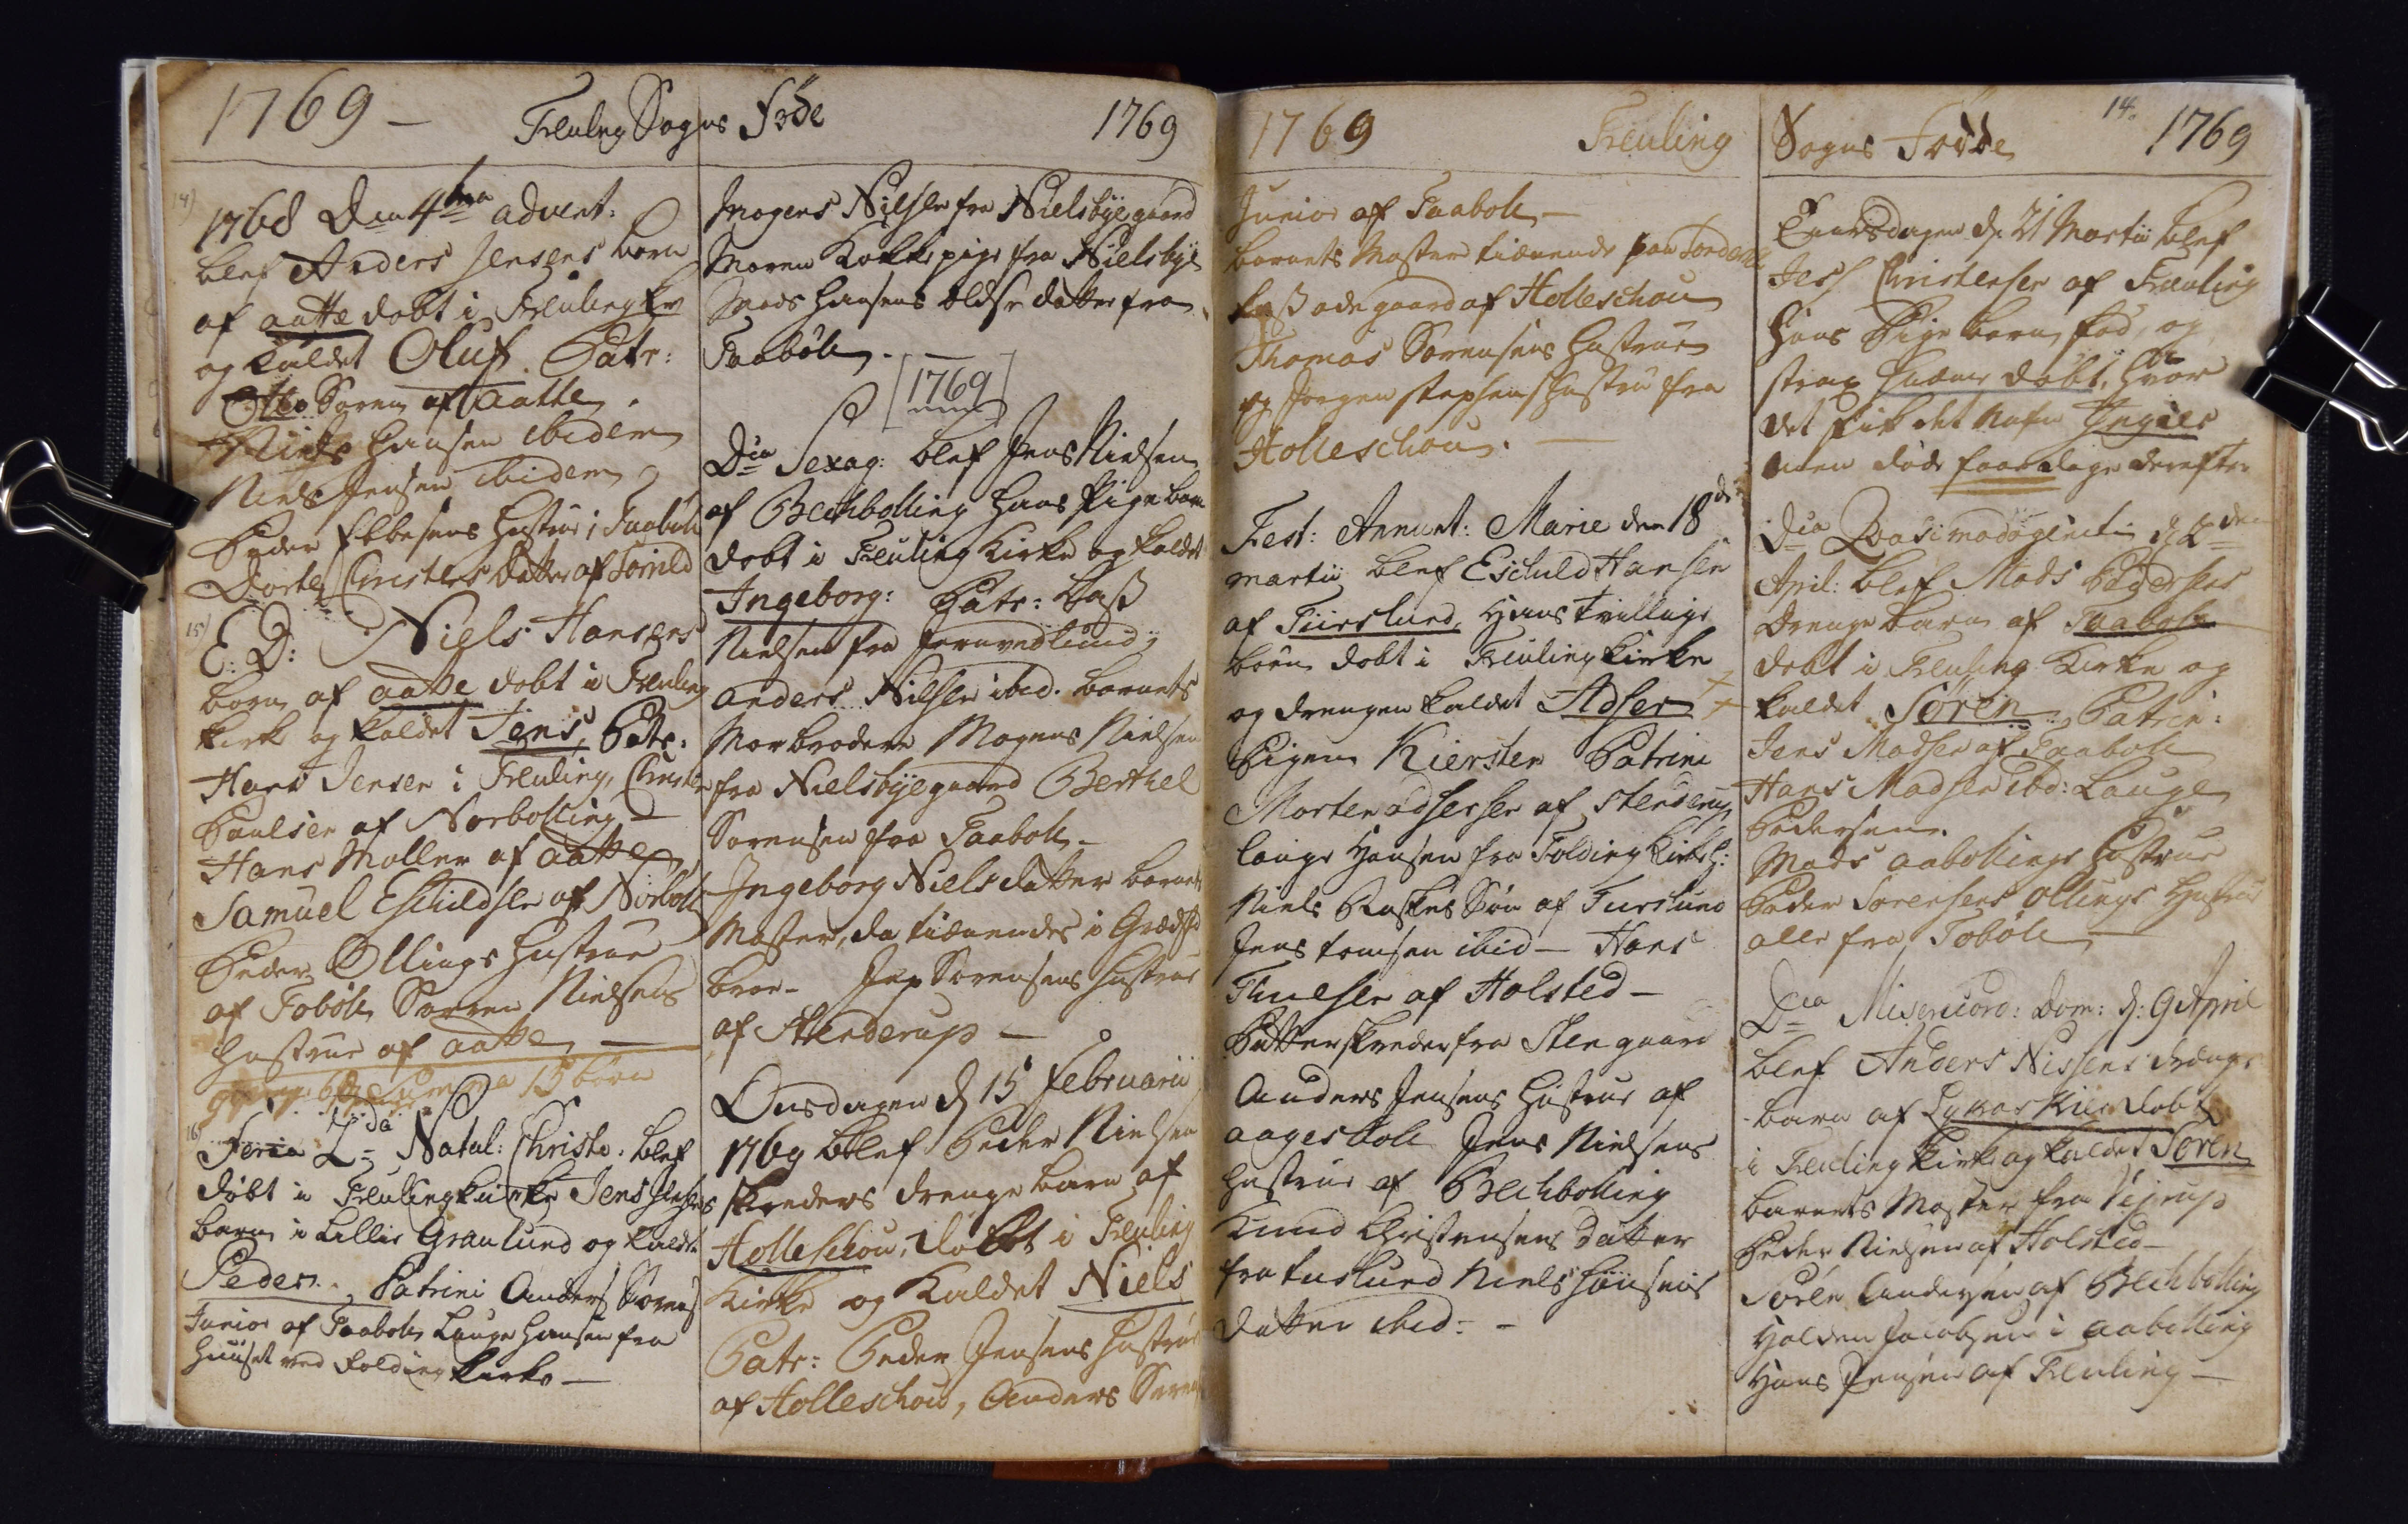1769
Feulings Sogns Føde
14)
1768 Dca 4tia Advent:
blev Anders Jensens barn
af Aatte døbt i Feuling Kr
og kaldet Oluf. Patr:
Otte Soren af Aatte
Niels Hansen ibidem
Niels Jensen ibidem
Peder Ebbesens Hustrue i Taabøll
Dorte Christens Datter af Sorrild
15
E: D: Niels Hansens
Barn af Aatte døbt i Feuling
Kirk og kaldet Jens Patr:
Hans Jensen i Feuling, Christen
Poulsen af Norbolling.
Hans Moller af Aatte
Samuel Eschildsen af Nørbolling
Peder Ollings Hustrue
af Tobøll Sorren Nielsens
Hustrue af Aatte
9 piger 6 drenge Summa 15 Børn
16)
Feria 2da Natal: Christi blef
døbt i Feuling Kirke Jens Jensens
barn i Lillie Graulund og kaldet
Peder. Patrini Anders Sørens
Junior af Taaboll Lauge Hansen fra
Huuset ved Folding Kirke -
1769
Mogens Nielsen og Nielsbyegaard
Maren Kokkepige fra Nielsbye
Mads Hansens ældse datter fra
Taabøll
1769
Dca Sexag: blef Jens Nielsen
af Bechbolling Hans Pige Barn
døbt i Feuling Kirke og kaldet
Ingeborg: Pater: Lass
Nielsen fra Jernvedlund
Anders Nielsen ibid: Barnets
Mor Broder Mogens Nielsen
fra Nielsbyegaard Berthel
Sorensen fra Taaboll
Ingeborg Niels datter barnets
Moster, da tienende i Grædsted
Broe. Jep Sørensens Hustrue
af Stenderup
Onsdagen d 15 Februarii
1769. blef Peder Nielsen
Skreders drenge Barn af
Holleschou,  døbt i Feuling
Kirke og kaldet Niels
Patr: Peder Jensens Hustrue
af Holleschou, Anders Sørens
1769
Feuling Sogns Fodde
Junior af Taaboll
Barnets Moster tienende paa ##
Lass odegaard af Holleschou
Thomas Sørensens Hustrue
og Jørgen Stephens Hustrue fra
Holleschou
Fest: Annunt: Marie den 18de
martii blef Eschild Hansen
af Tiirslund, Hans Tvillige
Børn dobt i Feuling Kirke
og Drengen kaldet Adser
Pigen Kiersten Patrini
Morten Adsersen af Stenderup
Lauge Hansen fra Folding Kirkeh:
Niels Raskes Søn af Tiirslund
Jens Tomsen ibid - Hans
Thuesen af Holsted -
P##er skredder fra Stengaard
Anders Jensens Hustrue af
aagesboll Jens Nielsens
Hustrue af Bechbolling
Knud Christensens Datter
fra tiislund Niels Hansens
datter ibid:
5
1769
Tiirsdagen d 21 Martii Blef
Jes Christensen af Feuling
hans Pige Barn fød, og
strax hieme døbt, hvor
det fik det Nafn Jngies
men døde faa##lige derefter
Dca Qvasimodogeniti d: 2den
April: Blef Mads Pedersens
Drenge Barn af Taaboll
døbt i Feuling Kirke og
kaldet Søren. Patrin:
Jens Madsen af Taaboll
Hans Madsen ibd: Lauge
Pedersen.
Mads aabollings Hustrue
Peder Sørensens Ollings Hustrue
alle fra Tobøll.
Dca Misericord: Dom: d 9 April.
Blef Anders Nissens Drenge
Barn af Lykaskier dobt
i Feuling Kirke og kaldet Søren
Barnets Moster fra Vejrup
Peder Nielsen af Holsted -
Søren Andersen af Bechbolling
Holden Jacobsen i aabolling
Hans Jensen af Feuling
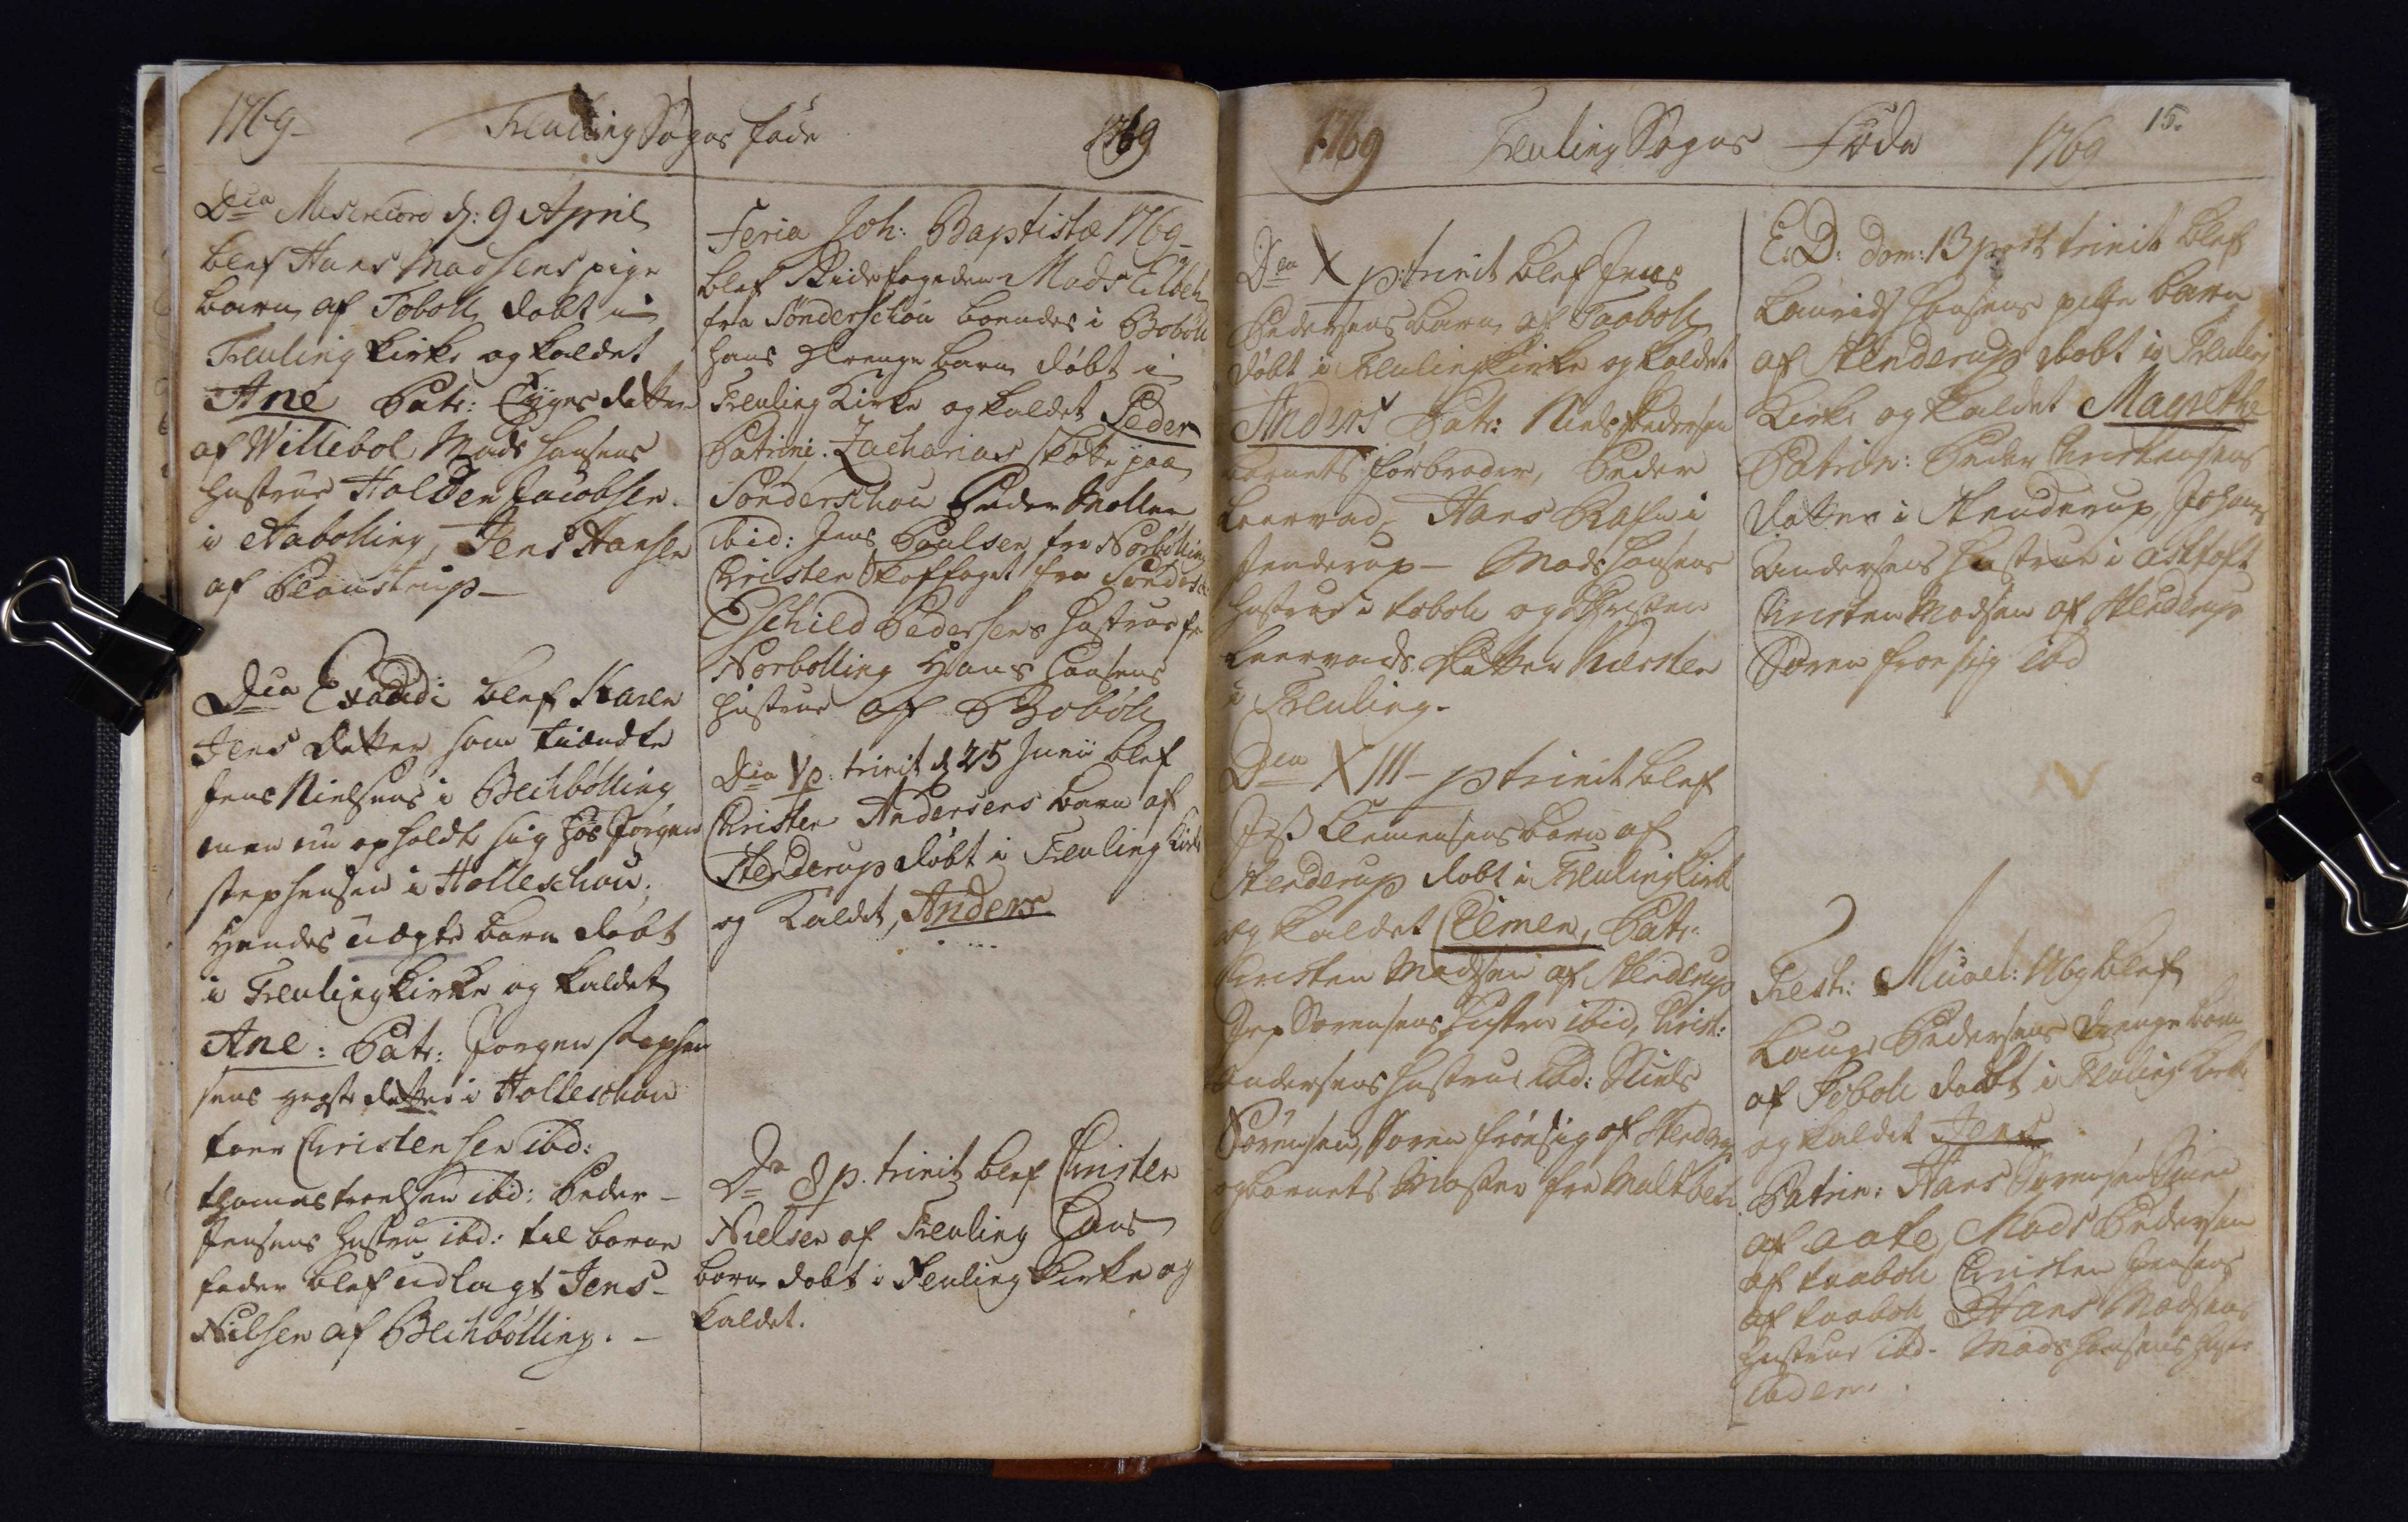1769.
Feuling Sogns føde
Dca Misericord d: 9 April
blef Hans Madsens pige.
Barn af Toboll døbt i
Feuling Kirke og kaldet
Ane Patr: Tygesdatter
af Willebol Mads Hansens
Hustrue Holden Jacobsen.
i Aabolling, Jens Hansen
af Ploustrup.
Dca Exaudi blef Karen
Jens Datter som tiændte
Jens Nielsens i Becbølling
men nu opholdt sig hos Jørgen
stephensen i Holleschou;
Hendes uægte Barn døbt
i Feuling Kirke og kaldet
Ane: Patr: Jørgen Stephen
sens yngste daatter i Holleschou
toer Christensen ibd:
Thomas Troelsen ibid: Peder -
Jensens Hustru ibd. til barne
fader blef udlagt Jens -
Nielsen af Bechølling.
1769
Feria Joh: Baptistæ 17609 -
blef Ridefogeden Mads Elbeh
fra Sønderschou boendes i Bobøll
hans Drenge Barn døbt i
Feuling Kirke og kaldet Peder
Patrini Zacharias skøtte paa
S'ønderschou Peder Moller
ibid: Jens Poulsen fra Norbølling
Christen Skoffoget fra Sonderschou
Eschild Pedersens Hustroe fr
Norbolling Hans Larsens
Hustrue af Bobøll
Dca V p: trinit d 25 Junii blef
Christen Andersens barn af
Stenderup døbt i Feuling Kirke
og kaldet Anders
Dca 8 p. trinit blef Christen
Nielsen af Feuling Hans
Barn døbt Feuling Kirke og
kaldet.
1769
Feuling Sogns
Dca X p: trinit blef Jens
Pedersens Barn af Taaboll
døbt i Feuling Kirke og kaldet
Anders Patr: Niels Pedersens
Barnets førbroder, Peder
Leervad Hans Nafn i
stenderup Mads Hansens
Hustrue boboll og Christen
Leervads Datter Kærsten
i Feuling.
Dca XIII - p Trinit blef
Jes Clemensens Barn af
Stenderup døbt i Feuling Kirke
og kaldet Clemen, Patr
Chresten Madsen af Stenderup
Jep Sørensens Hustru ibid, Christ:
Andersens Hustrue ibid: Niels
Sørensen, Søren Frøsig af Stenderup
og Barnets Moster fra Maltbech
15
Føde
1769
E: D: Dom: 13 post Trinit blev
Laurids Hansens pige barn
af Stenderup døbt i Feuling
Kirke og kaldet Magrethe
Patrin: Peder Christensens
Datter i Stenderup, Johannes
Andersens Hustrue i Atoft
Christen Madsen af Stenderup
Søren Froesig ibd.
Fest. Michel ##bg blef
Lauge Pedersens Drenge Barn
af Toboll døbt i Feuling Kirke
og kaldet Jens
Patrin: Hans Sorensen Smed
af aate Mads Pedersen
af taaboll Christen Jensens
af taaboll Hans Madsens
Hustrue ibid: Mads Hansens Hustr
ibdem